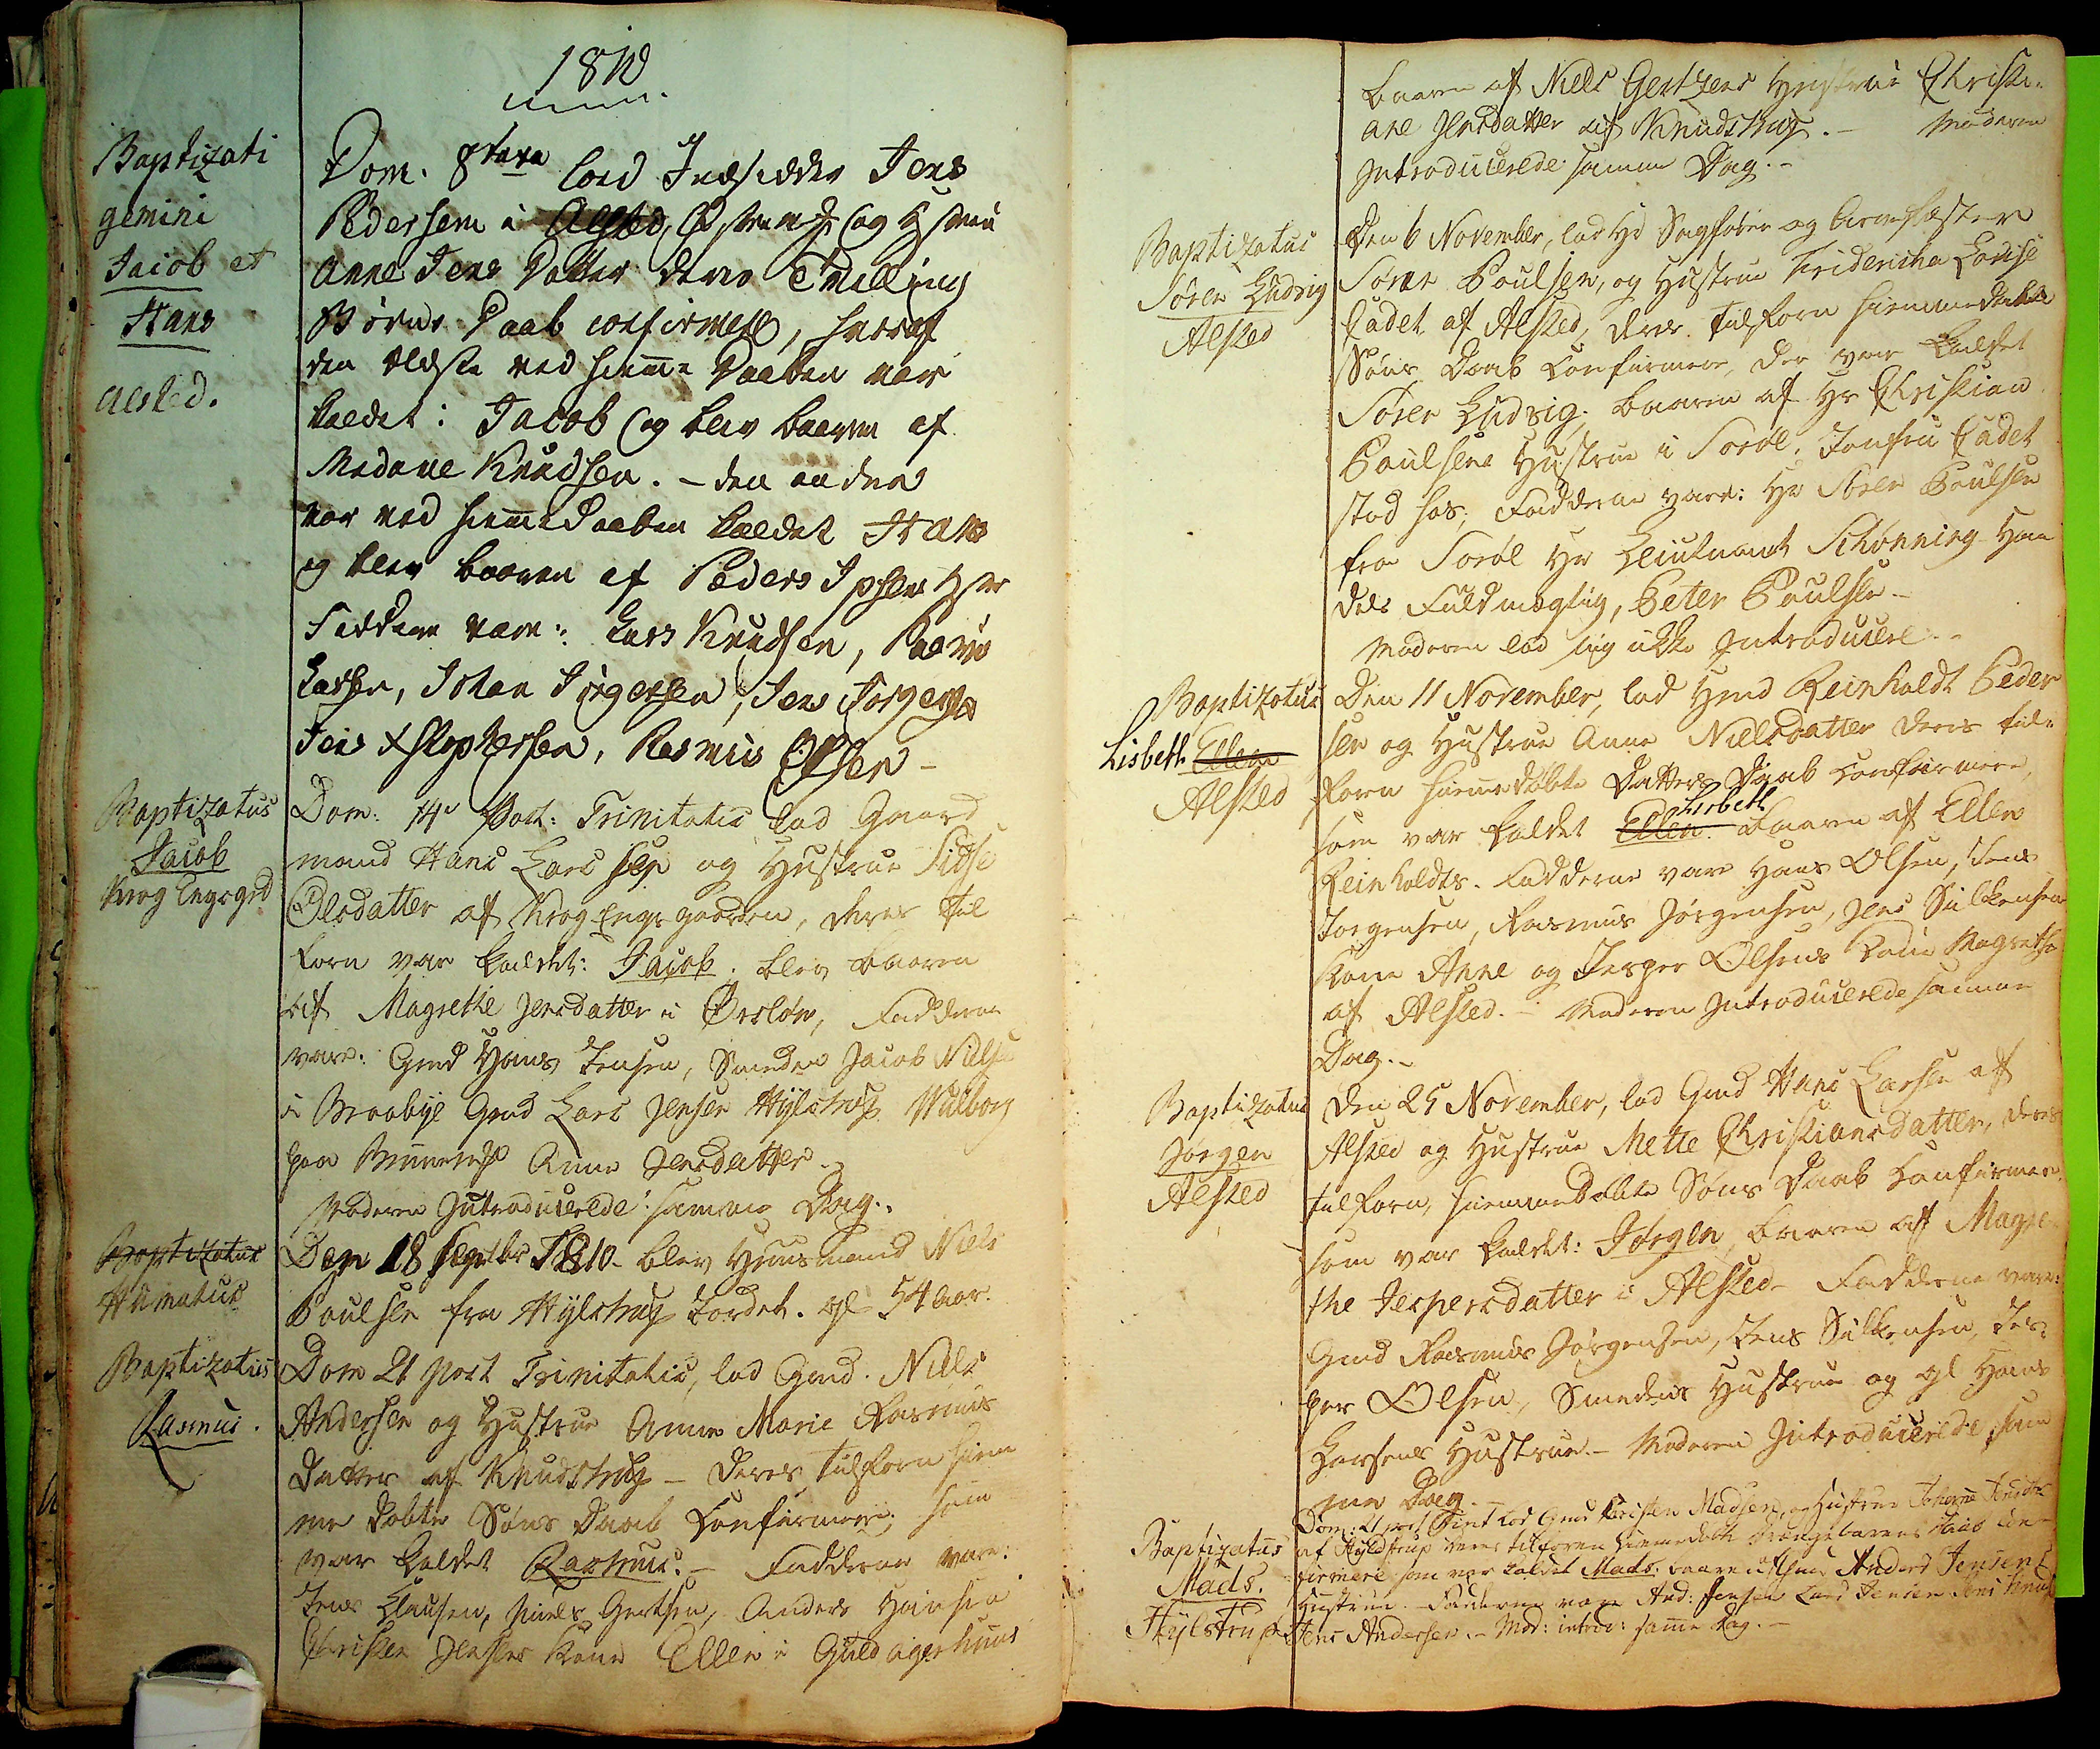Baptizati
gemini
Jacob et
Hans
Alsted.
Baptizatus
Jacob
Krog ##eg##grd
Baptizatus
Humatrus
Baptizatus
Rasmus
1810
Dom: 8ta## loed Indsidder Jens
Pedersen i Alsted Østrem## og Hstru
Anne Jens Datter, deres Tvilling
Børns Daab confirmere, hvoraf
den ældste ved hiemme Daaben var
kaldet: Jacob og blev baaren af
Madame Knudsen. - den anden
var ved hiemmedaaben kaldet Hans
og blev baaren af Peder Ipsens Hstr
Faddere vare: Lars Knudsen, Rasmus
Lassen, Johan Jørgenssen, Jens Jørgensen
Jens Xstopfersen, Rasmus Olsen
Dom: 14 Post: Trinitatis lod Gaard
mand Hans Larsen og Hustrue Sidse
Olsdatter af Krog Engsgaarden, deres til
forn var kaldet: Jacob. blev baaren
af Magrethe Jensdatter i Ørslev, Faddere
var: Gmd Hans Jensen, Smeden Jacob Nielsen
i Braabye Gmd Lars Jensen Hylstrup Walborg
paa Brunem## Anne Jensdatter.
Moderen Introducerede samme Dag.
Den 18 Septbr 1810. blev Huusmand Niels
Poulsen fra Hylstrup Jordet. gl 54 Aar
Dom 21 post Trinitatis lod Gmd. Niels
Andersen og Hustrue Anne Marie Rasmus
datter af Knudstrup - deres tilforn hiem
me døbte Søns Daab Confirmere; som
var kaldet Rasmus. Fadderne vare:
Jens Clausen, Niels Gertsen, Anders Hansen
Christen Jensens Kone Ellen Guldagerhuus
Baptizatus
Søren Ludvig
Alsted
Baptixatus
Lisbeth Ellen
Alsted
Baptizatus
Jørgen
Alsted
Baptizatus
Mads
Hylstrup
baaren af Niels Gertsens Hustrue Christi¬
ane Jensdatter af Knudstrup. - Moderen
Introducerede samme Dag.
Den 6 November, lod Hr Sagførerr og Arvefæster
Søren Poulsen, og Hustrue Fridericha Louise
Cadet af Alsted, deres tilforn hiemmedøbte
Søns Daab Confirmere, der var kaldet
Søren Ludvig. baaren af Hr Christian
Poulsens Hustrue i Sorøe. Jomfru Cadet
stod hos, Faddere vare: Hr Søren Poulsen
fra Sorøe Hr Leutenant Schønning. Han
dels Fuldmægtig, Peter Poulsen
Moderen lod sig ikke Introducere
Den 11 November lod Hmd Reinholdt Peder
sen og Hustrue Anne Nielsdatter deres tie¬
forn hiemmedøbte Datters Daab Confirmere
Lisbeth
som var kaldet Ellen. baaren af Ellen
Reinholdts. Fadderne vare Hans Olsen, Jens
Jørgensen, Rasmus Jørgensen, Jens Silkensens
Kone Anne og Jesper Olsens Kone Magrethe
af Alsted. - Moderen Introducerede samme
Dag. -
den 25 November, lod Gmd Hans Larsen af
Alsted og Hustrue Mette Christiansdatter, deres
tilforn hiemmedøbte Søns Daab Confirmere
som var kaldet: Jørgen baaren af Magre¬
the Jespersdatter i Alsted. Fadderne vare:
Gmd, Rasmus Jørgensen, Jens Silkensen, Jes¬
per Olsen, Smedens Hustrue og gl Hans
Larsens Hustrue. - Moderen Introducerede, sam
me Dag.
Dom: 21 post Trint lod Gmd Christen Madsen og Hustrue Johanne Jensdtr
af Hylstrup deres tilforn hiemmedøbte Drængebarns Daab con¬
af
firmere som var kaldet Mads, baaren Hmd Anders Jensens
Hustrue. Fadderne vare And: Jensen Lars Jensen Jens Knuds
Jens Andersen. Mod: introd: samme Dag.
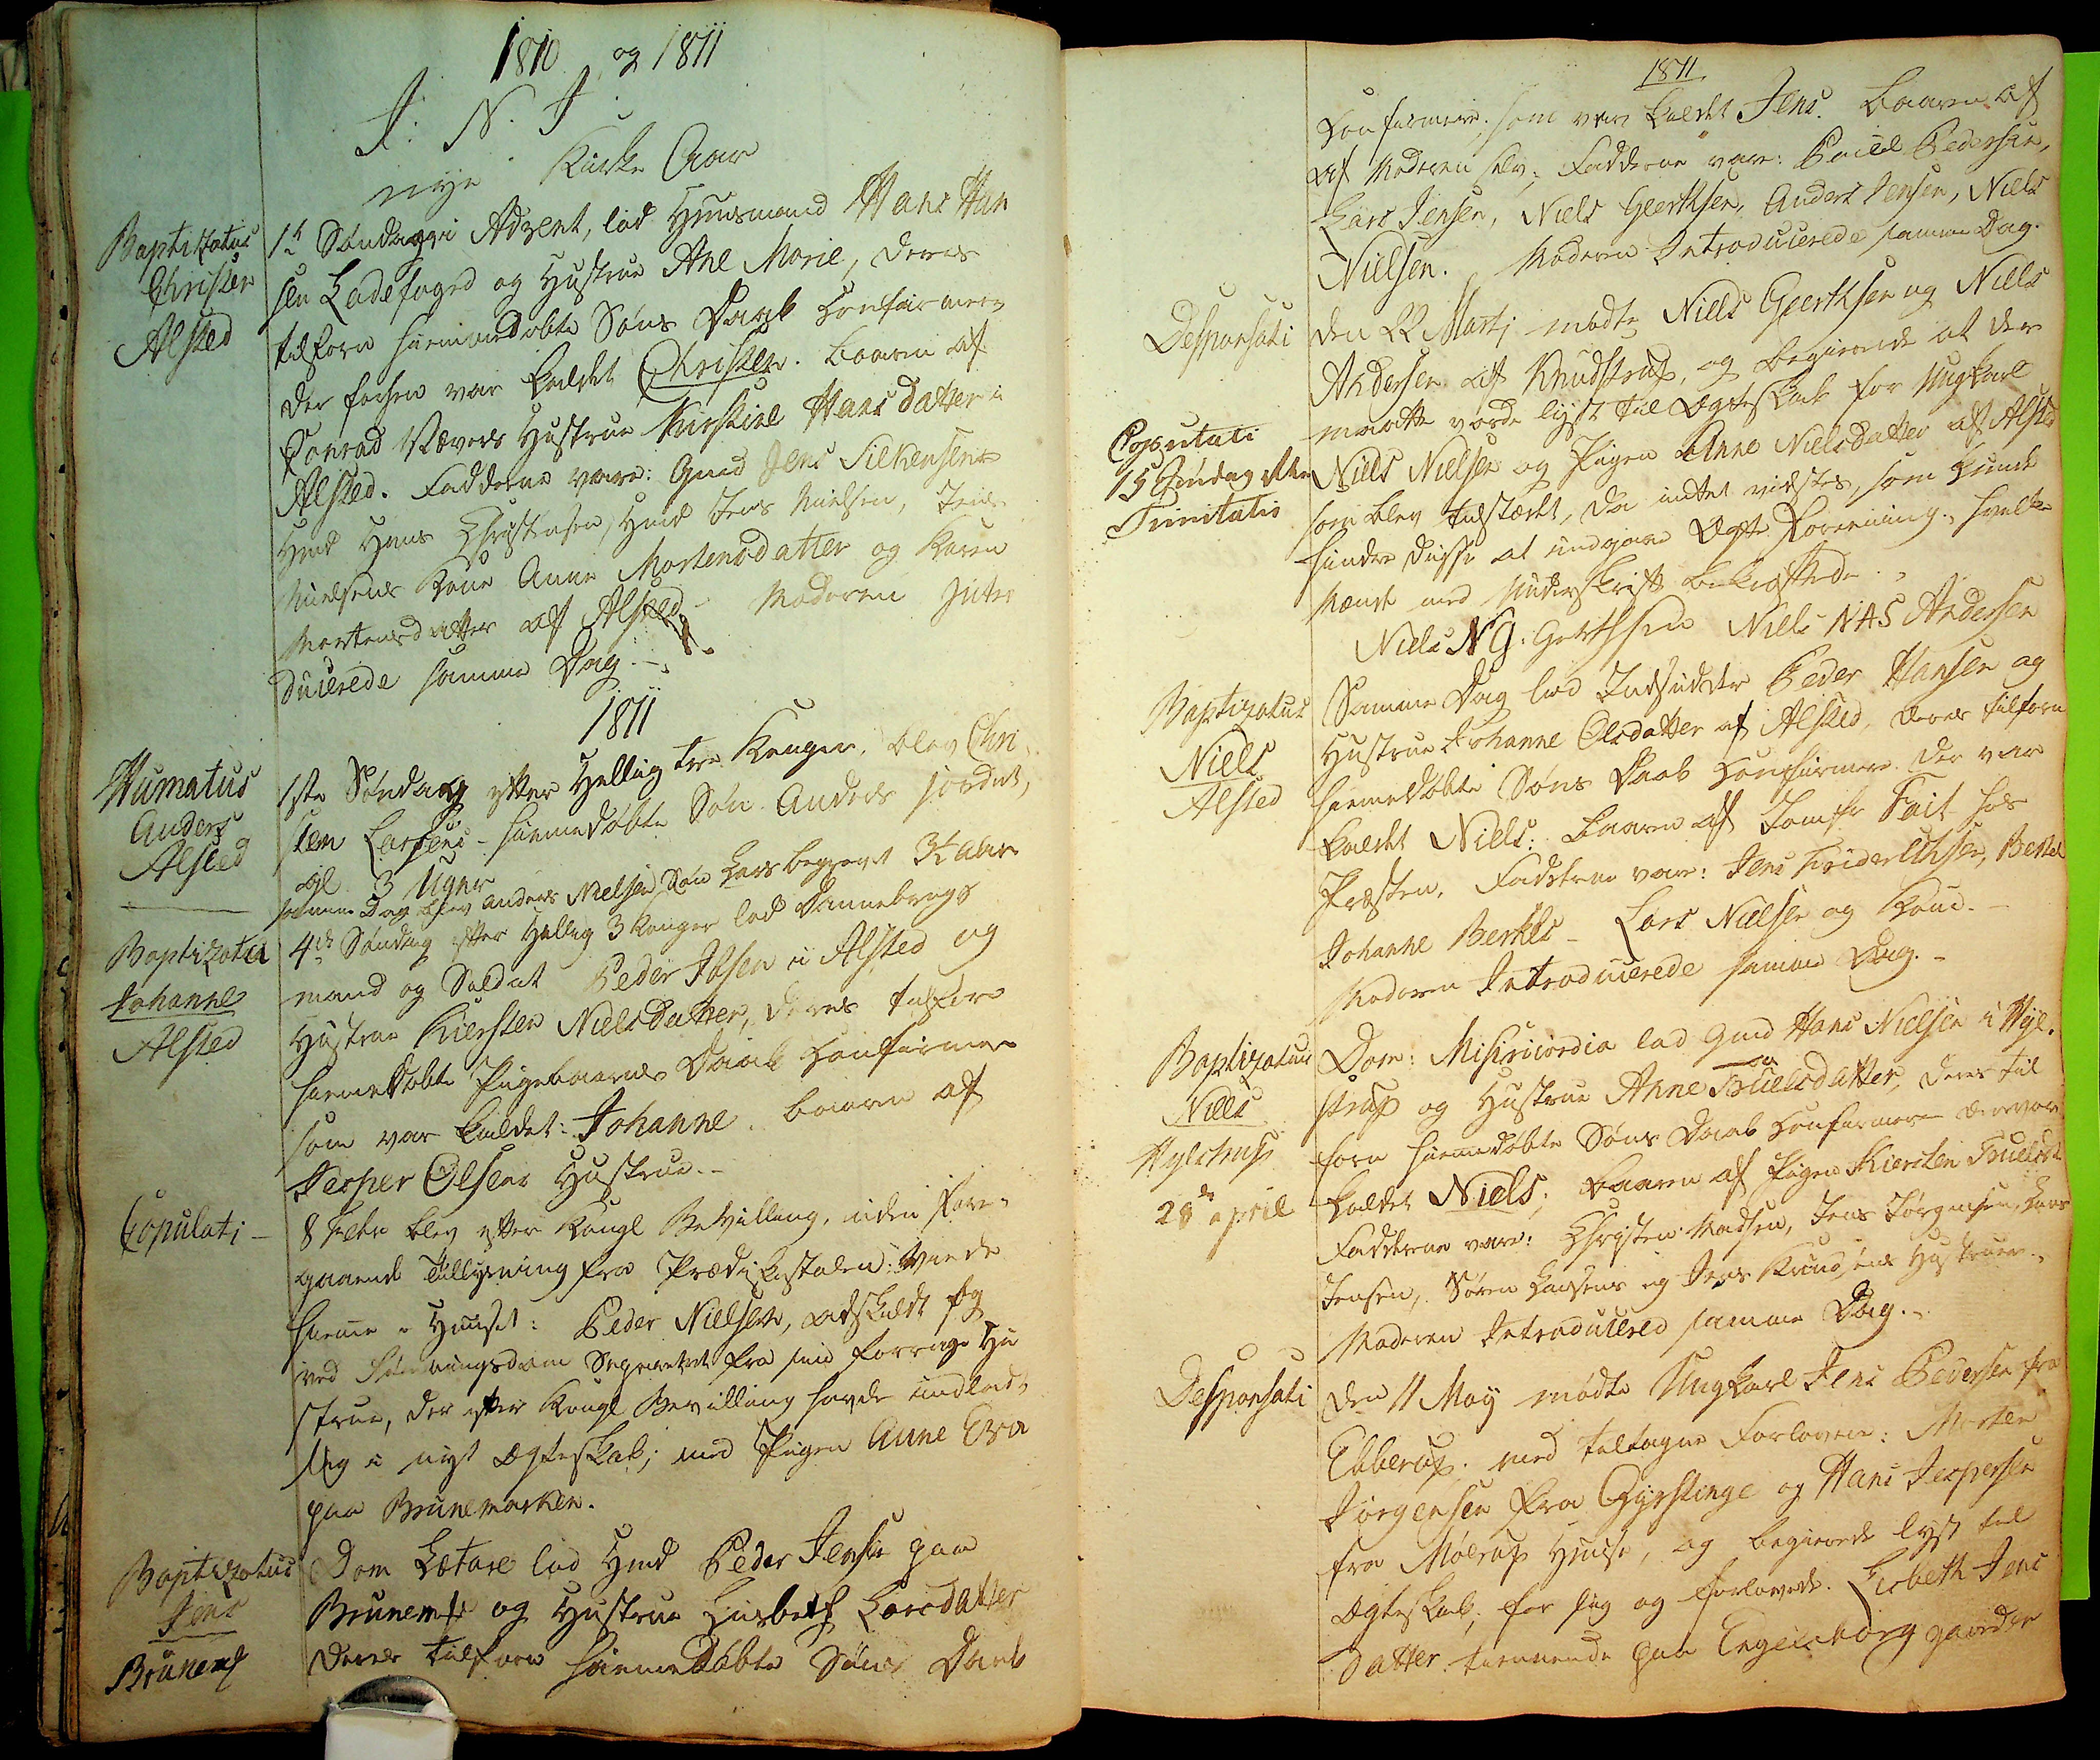Baptizatus
Christen
Alsted
Humatus
Anders
Alsted
Baptizata
Johanne
Alsted
Copulati
Baptizatus
Jens
Brunem##
1810 og 1811
J: N: J:
nye Kirke Aar
1te Søndag i Advent, lod Huusmand Hans Han
sen Ladefoged og Hustrue Ane Marie, deres
tilforn hiemmedøbte Søns Daab Confirmere
der forhen var kaldet Christen. baaren af
Conrad Vævers Hustrue Kirstine Hansdatter i
Alsted. Fadderne vare: Gmd Jens Silkenens
Hmd Hans Christensen Hmd Jens Nielsen, Jens
Nielsens Kone Anne Mortensdatter og Karen
Mortensdatter af Alsted, Moderen Intro
ducerede samme Dag.
1811
1ste Søndag efter Hellig tre Konger blev Chri¬
sten Larsens hiemmedøbte Søn Anders jordet,
gl: 3 Uger
Samme Dag blev Anders Nielsen Søn Lars begravet 3½ Aar
4de Søndag efter Hellig 3 Konger lod Dannebrogs
mand og Soldat Peder Ibsen i Alsted og
Hustrue Kiersten Nielsdatter, deres tilforn
hiemmedøbte, Pigebarns Daab Confirmere
som var kaldet: Johanne. baaren af
Jesper Olsens Hustrue.
8 Febr blev efter Kongl Bevilling, uden fore
gaaende Tillysning fra Prædikestolen. Viede
hiemme i Huuset: Peder Nielsen, adskildt
ved S##ingsdom Se##t fra sin forrige Hu
strue, der efter Kongl Bevilling havde indladt
sig i nyt Ægteskab; med Pigen Anne Eva
paa Brunemarken.
Dom Lætare lod Hmd Peder Jensen paa
Brunem## og Hustrue Lisbeth Larsdatter
deres tilforn hiemmedøbte Søns Daab
Desponsati
Copulati
15 Søndag efter
Trinitatis
Baptizatus
Niels
Alsted.
Baptizatus
Niels
Hyklstrup.
28de April
Desponsati
1811
Confirmere som var kaldet Jens. Baaren af
af Moderen selv. Fadderne vare Poul Pedersen
Lars Jensen, Niels Geertsen, Anders Jensen, Niels
Nielsen. Moderen Introducerede samme Dag.
den 22 Martj mødte Niels Geertsen og Niels
Andersen af Knudstrup, og begierede at der
maatte vorde lyst til Ægteskab for Ungkarl
Niels Nielsen og Pigen Anne Nielsdatter af Alsted
som blev tidstædet, da intet vidstes, som kunde
hindre disse, at indegaae Ægte Forening, hvilke
Mænd med Underskrift be##
Niels N G Geertsen Niels N A S Andersen
Samme Dag lod Indsidder Peder Hansen og
Hustrue Johanne Olsdatter af Alsted, deres tilforn
hiemmedøbte Søns Daab Confirmere, der var
kaldet Niels: baaren aff Jomfr Fait hos
Præsten, Fadderne vare: Jens Friderichsen, Bertel
Johanne Bertels - Lars Nielsen og Kone
Moderen Introducerede samme Dag.
Dom: Misericordia lod Gmd Hans Nielsen i Hyl¬
strup og Hustrue Anne Truelsdatter, deres til
forn hiemmedøbte Søns Daab Confirmere der var
kaldet Niels. baaren af Pigen Kiersten Truelsdtr
Fadderne vare: Christen Madsen, Jens Jørgensen, Hans
Jensen, Søren Hansens og Jens Knudsens Hustruer.
Moderen Introducered samme Dag.
dend 11 Maij mødte Ungkarl Jens Pedersen fra
Ebberup, med tilligen Forlover: Morten
Jørgensen fra Gyrstinge og Hans Jespersen
fra Mølrup Huuse, og begierede lyst til
Ægteskab, for sig og Forlovede. Lisbeth Jens
Datter tiennende paa Engelborg Gaarden